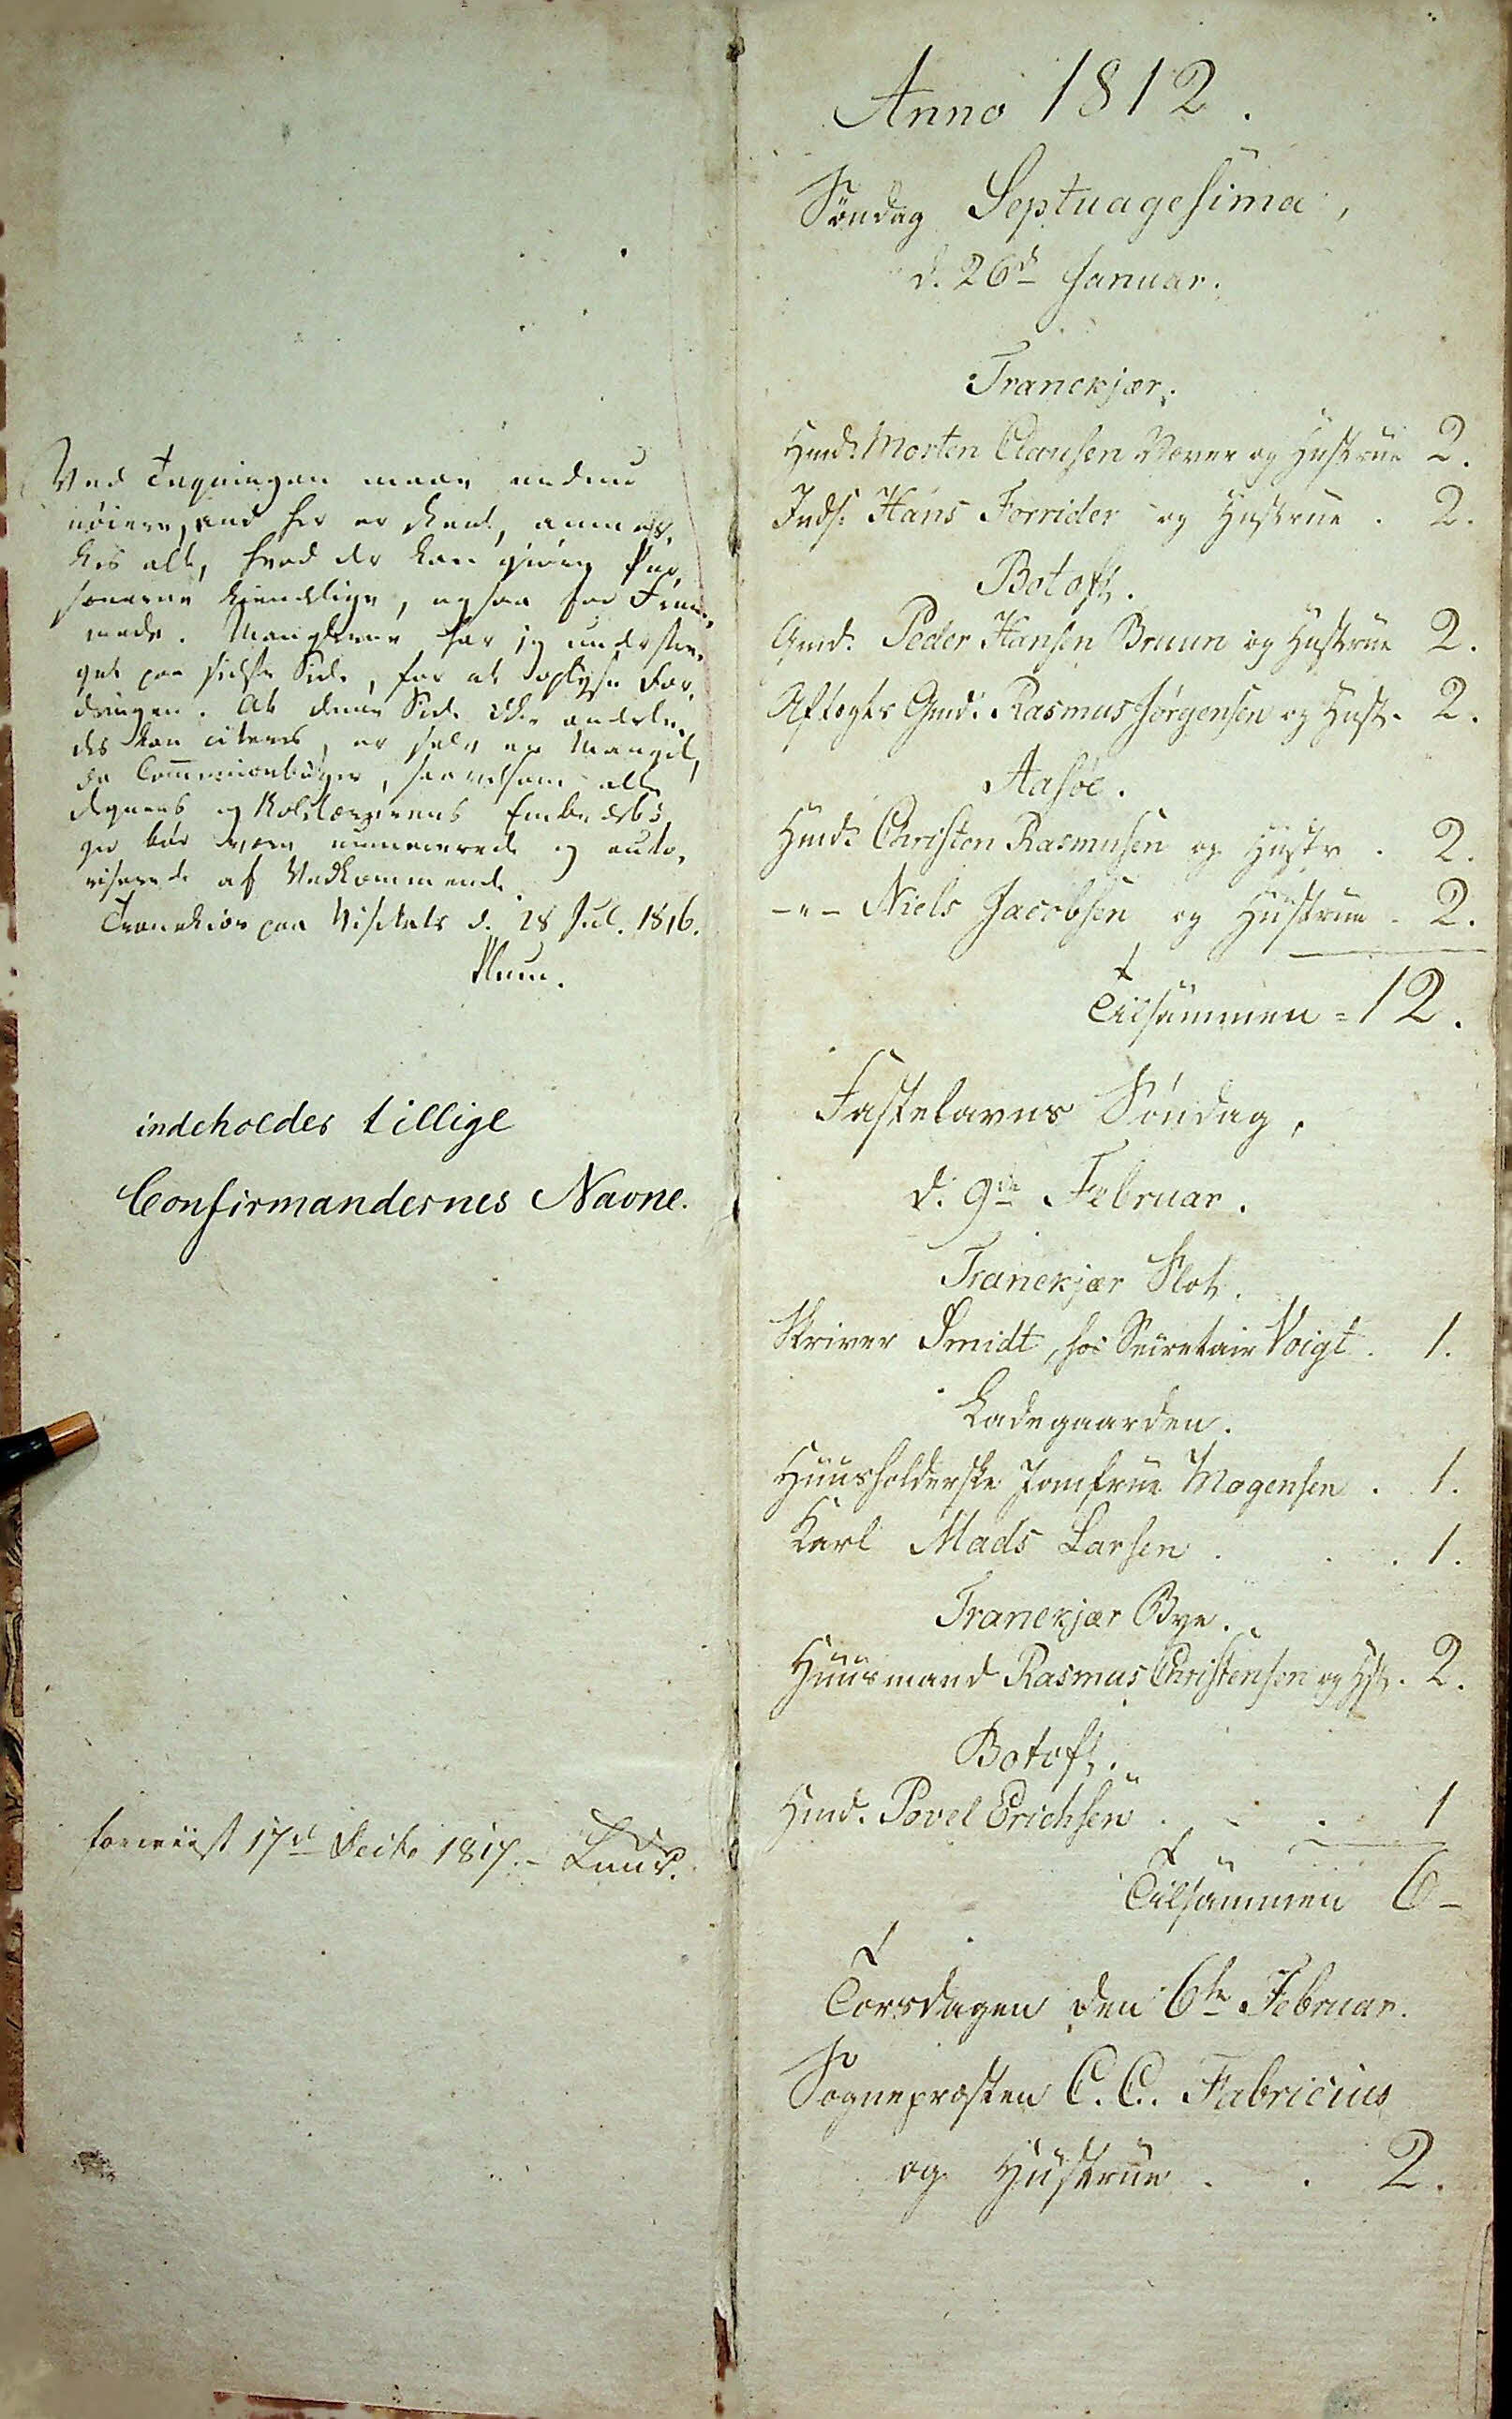Med Tegningen maae unden
nøiere, ved her er kent, anning¬
Kis alt, hvad de kan ## Per¬
sonerne kiendelige, ogsaa for Frem¬
mede. Manglerne har ieg understre¬
get paa sidste Side, for at oplyse For¬
dringen. At denne Side ## anderle¬
des kan citeres, og selv er mangel¬
de Communionbogen, saa velsom alle
degnens og Kollængens ## ##
## bør ## nummmerde og auto¬
riserede af Vedkommende
Tranekiær paa Visitats d. 28 Jul. 1816.
##
indholdes tillige
Confirmandernes Navne.
foreviist 17de  Decbr 1817. - Lund.
Anno 1812
Søndag Septuagesima
d. 26de Januar:
Tranrkjær
Hmd: Morten Clausen Væver og Hustrue 2.
Inds: Hans Forrider og Hustrue . 2.
Botoft.
Grd: Peder Hansen Bruun og Hustrue 2.
Aftegts Gmd. Rasmus Jørgensen og Hust . 2.
Aasøe.
Hmd. Christen Rasmusen og Hustr . 2.
-"- Niels Jacobsen og Hustrue . 2.
Tilsammen = 12.
Fastelavns Søndag
d: 9de Februar.
Tranekjær Slot.
Skriver Smidt, hos Seiretair Voigt . 1.
Ladegaarden.
Huusholderske Jomfrue Mogensen . 1.
Karl Mads Larsen . . . 1.
Tranekjær Bye.
Huusmand Rasmus Christensen og Hst . 2.
Botoft
Hmd. Povel Erichsens . . . 1
Tilsammen 6
Torsdagen den 6te Februar
Sognepræsten C. C. Fabriucius
og Hustrue . . 2.
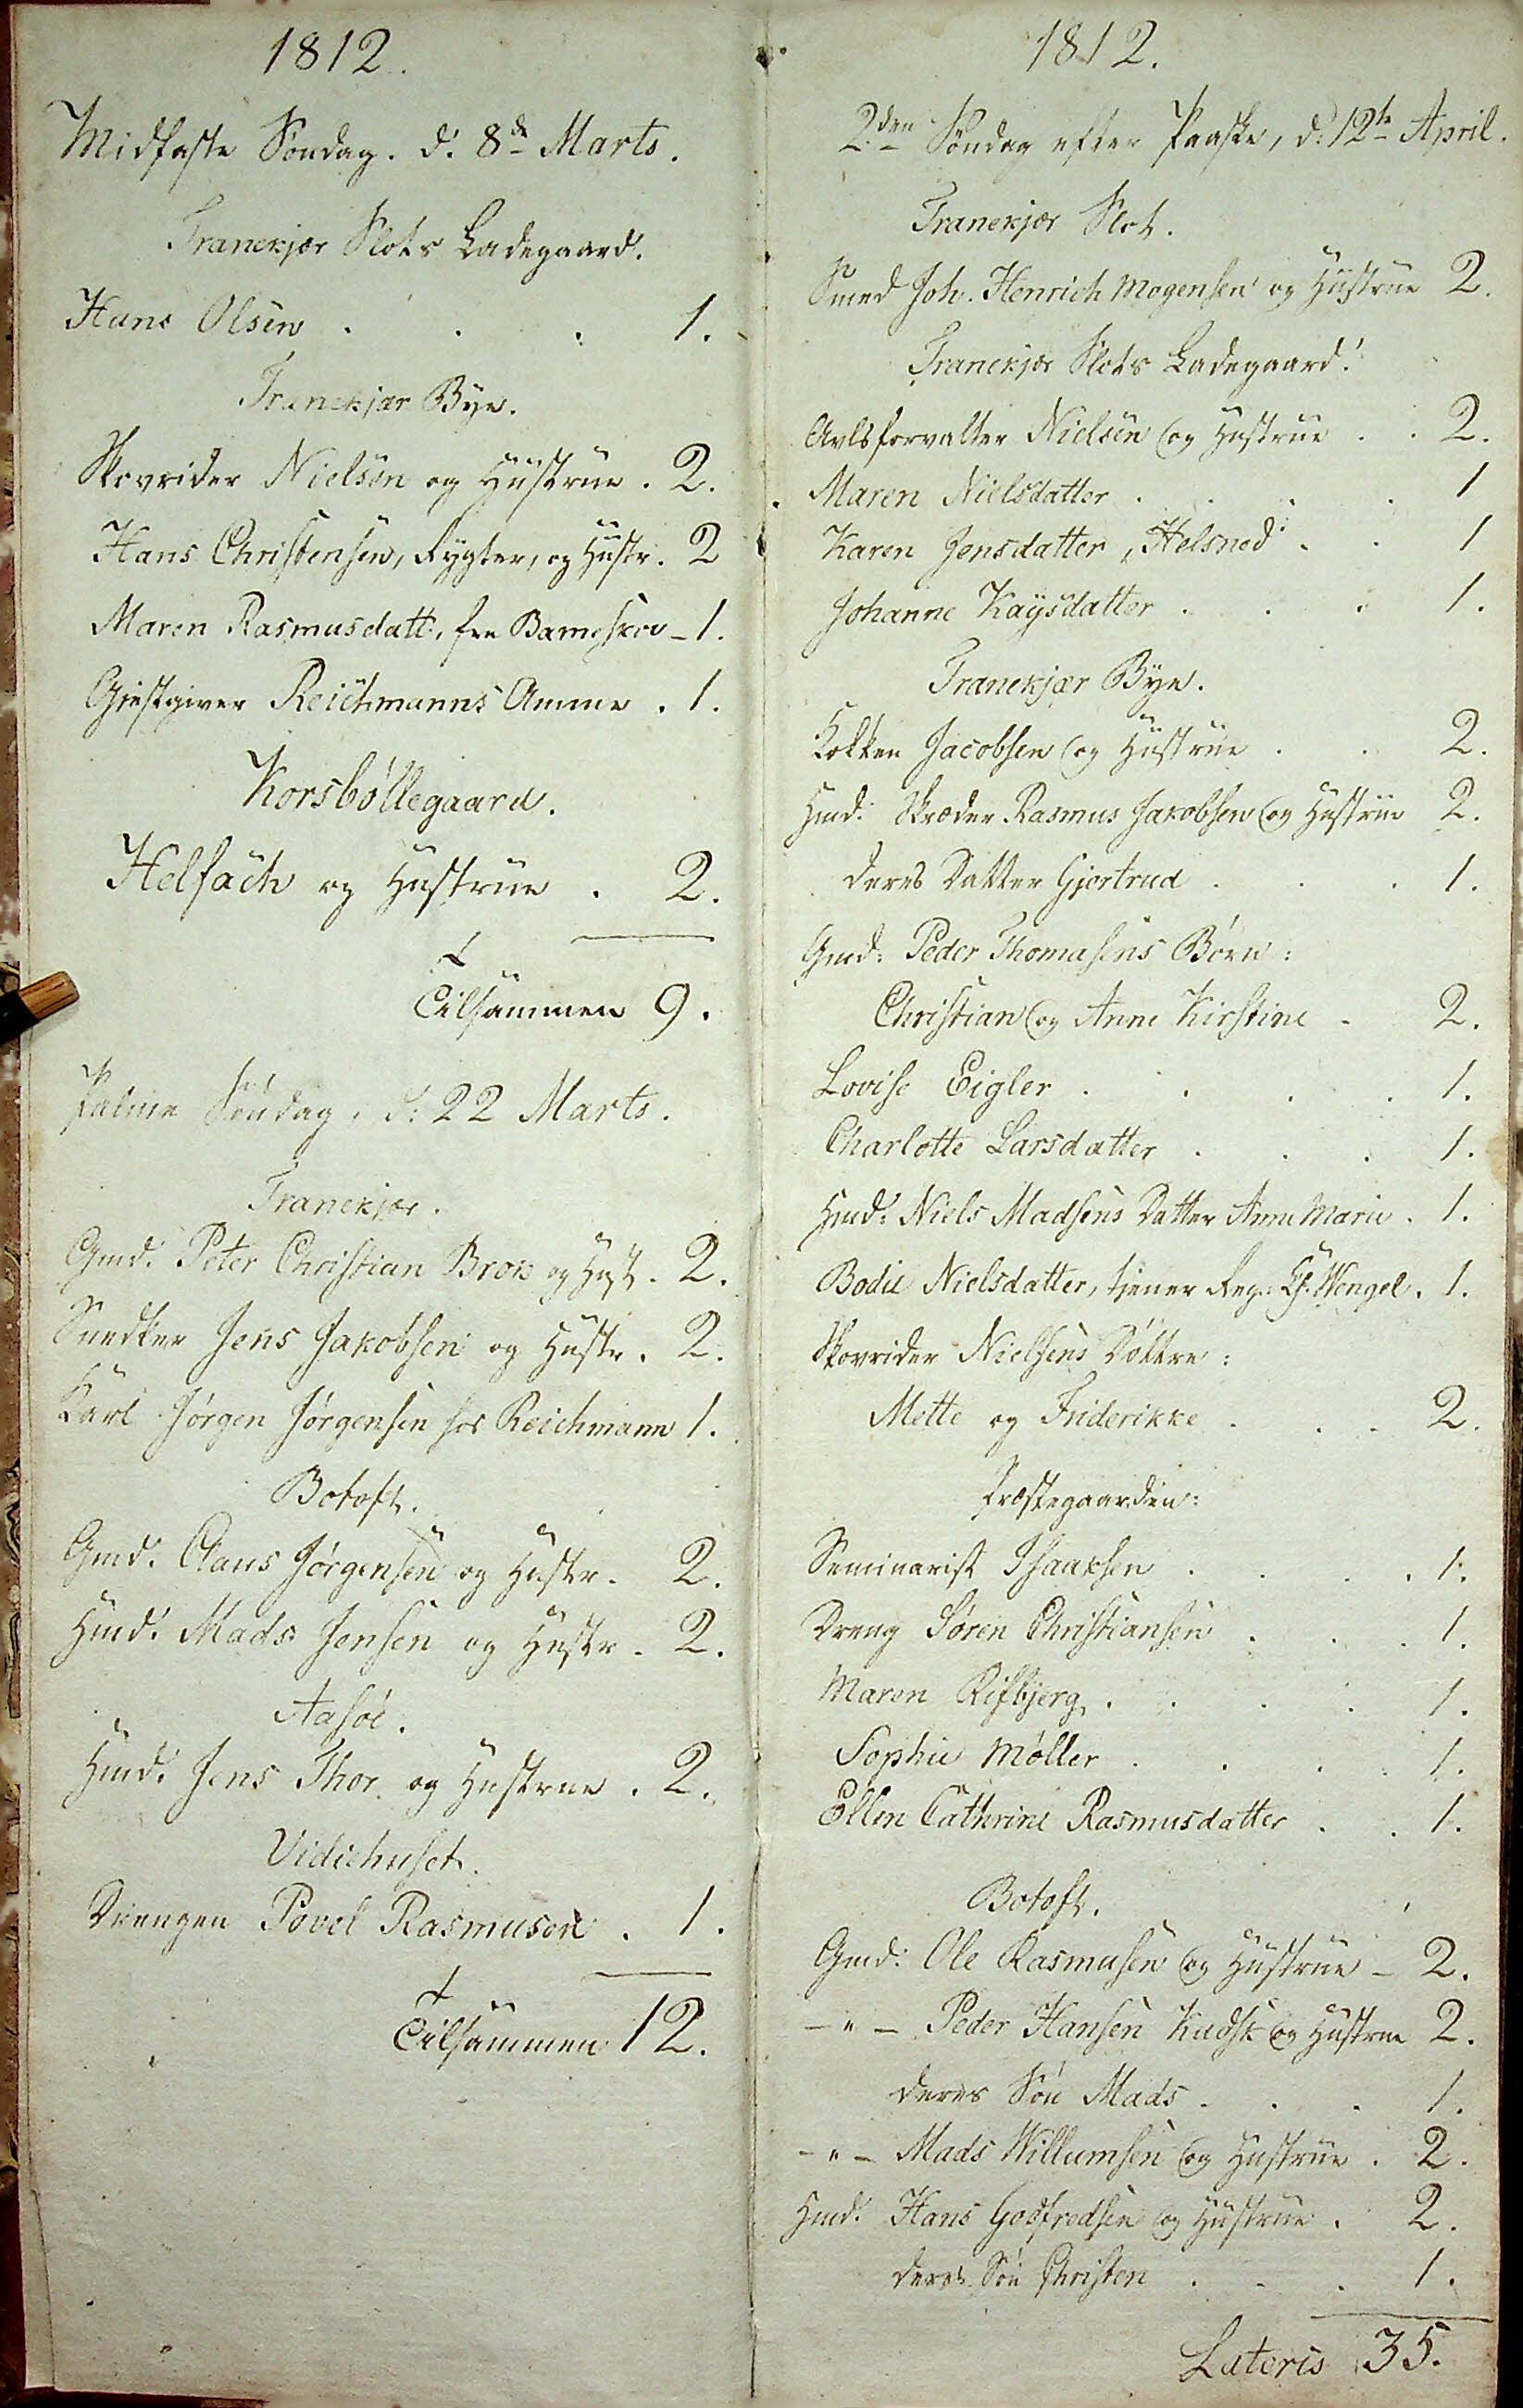1812.
Midfaste Søndag. d. 8de Marts.
Tranekjær Slots Ladegaard.
Hans Olsen . . . 1.
Tranekjær Bye.
Skovrider Nielsen og Hustrue . 2.
Hans Christensen Rygter, og Hustr . 2.
Maren Rasmusdatt, fra Bameskov - 1.
Giestgiver Reichmanns Amme . 1.
Korsbøllegaard.
Helfach og Hustrue . 2.
Tilsammen 9.
Palme Søndag d: 22 Marts.
Tranekjær.
Gmd. Pater Christian Brok og Hust . 2.
Snedker Jens Jacobsen og Hustr . 2.
Karl Jørgen Jørgensen hos Reichmann 1.
Botoft.
Gmd. Claus Jørgensen og Hustr . 2.
Hmd. Mads Jensen og Hustr . 2.
Aasøe.
Hmd. Jens Thor og Hustrue . 2.
Vidiehuset
Drengen Povel Rasmuson . 1.
Tilsammen 12.
1812.
2den Søndag efter Paaske, d: 12te April.
Tranekjær Slot.
Smed Johan Henrich Mogensen og Hustrue 2.
Tranekjær Slots Ladegaard.
Avlsforvalter Nielsen og Hustrue . . 2.
Maren Nielsdatter . . . . 1
Karen Jensdatter Helsned . . 1
Johanne Kaysdatter . . . 1.
Tranekjær Bye.
Kokken Jacobsens og Hustrue . . 2.
Hmd: Skræder Rasmus Jacobsens og Hustrue 2.
deres Datter Giertrud . . . 1.
Gmd: Peder Thomasens Børn:
Christian og Anne Kirstine . 2
Lovise Eigler . . . . 1.
Charlotte Larsdatter . . . 1.
Hmd: Niels Madsens Datter Anne Marie . 1.
Bodil Nielsdatter, tjener Reg: Ch.: Wengel . 1.
Skovrider Nielsens Døttre :
Mette og Friderikke . . .  2.
Præstegaarden:
Seminarist Isaaksen . . . . 1.
Dreng Søren Christiansen . . . 1.
Maren Rifberg . . . . 1.
Sophie Møller . . . . 1.
Ellen Cathrine Rasmusdatter . . 1.
Botoft.
Gmd: Ole Rasmusen og Hustrue - 2.
-"- Peder Hansen Kudsk og Hustrue 2.
deres Søn Mads . . . 1.
-"- Mads Willumsen og Hustrue . 2.
Hmd. Hans Godfredsen og Hustrue . 2
deres Søn Christen . . . 1.
Lateris 35.